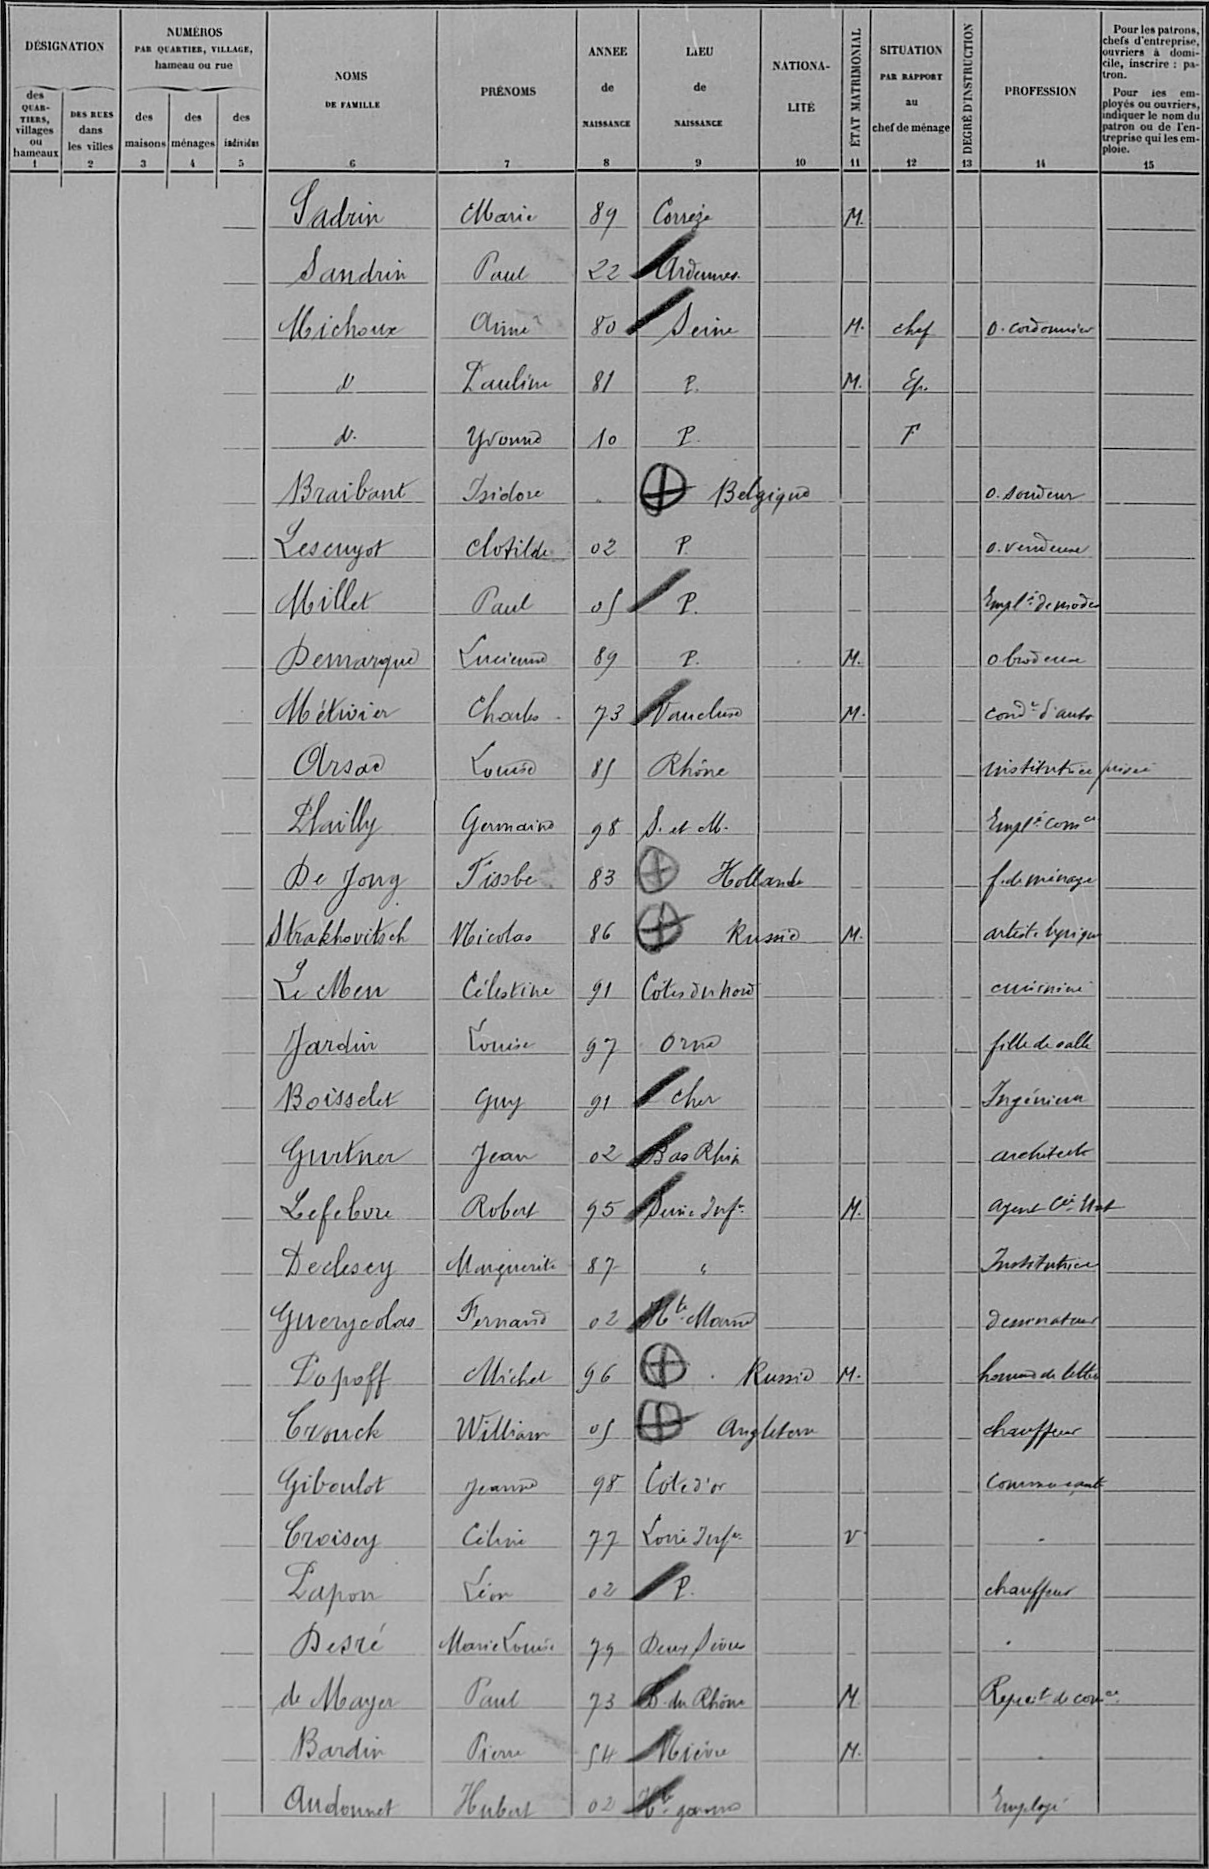Sadrin/Marie/89/Corrèze/¤/M /¤/¤/¤/¤
Sandrin/Paul/22/Ardennes/¤/¤/¤/¤/¤/¤
Michoux/Aimé/80/Seine/¤/M /chef/¤/O Cordonnier/¤
d°/Pauline/81/P /¤/M /Ep /¤/¤/¤
d°/Yvonne/10/P/¤/¤/F/¤/¤/¤
Braibant/Isidore/¤/Belgique/¤/¤/¤/¤/o soudeur/¤
Lescuyot/Clotilde/02/P/¤/¤/¤/¤/o vendeuse/¤
Millet/Paul/05/P /¤/¤/¤/¤/Empl de mode/¤
Demarque/Lucienne/89/P /¤/M /¤/¤/o brodeuse/¤
Métivier/Charles/73/Vaucluse/¤/M /¤/¤/cond d'auto/¤
Arsac/Louise/85/Rhône/¤/¤/¤/¤/institutrice privée/¤
Dhailly/Germaine/98/S et M/¤/¤/¤/¤/Emplé comce/¤
De Jong/Fissbe/83/Hollande/¤/¤/¤/¤/f de ménage/¤
Strakhovitsch/Nicolas/86/Russie/¤/M/¤/¤/artiste lyrique/¤
Le Meu/Célestine/91/Cotes du Nord/¤/¤/¤/¤/cuisinière/¤
Jardin/Louise/97/Orne/¤/¤/¤/¤/fille de salle/¤
Boisselet/Guy/91/Cher/¤/¤/¤/¤/Ingénieur/¤
Gurtner/Jean/02/Bas Rhin/¤/¤/¤/¤/architecte/¤
Lefebvre/Robert/95/Seine Inf/¤/M /¤/¤/agent Cie Nat/¤
Declesey/Marguerite/87/¤/¤/¤/¤/¤/Institutrice/¤
Guerycolas/Fernand/02/Hte Marne/¤/¤/¤/¤/dessinateur/¤
Dopoff/Michel/96/Russie/¤/M /¤/¤/homme de lettres/¤
Crouch/William/05/Angleterre/¤/¤/¤/¤/chauffeur/¤
Giboulot/Jeanne/98/Cote d'or/¤/¤/¤/¤/Commerçant/¤
Croisy/Céline/77/Loire Infr/¤/V/¤/¤/¤/¤
Lapon/Léon/02/P/¤/¤/¤/¤/chauffeur/¤
Desré/Marie Louise/79/Deux Sèvres/¤/¤/¤/¤/¤/¤
de Mayer/Paul/73/B du Rhône/¤/M/¤/¤/Rept de comce/¤
Bardin/Pierre/54/Nièvre/¤/M /¤/¤/¤/¤
Audonnet/Hubert/02/Hte Garonne/¤/¤/¤/¤/Employé/¤
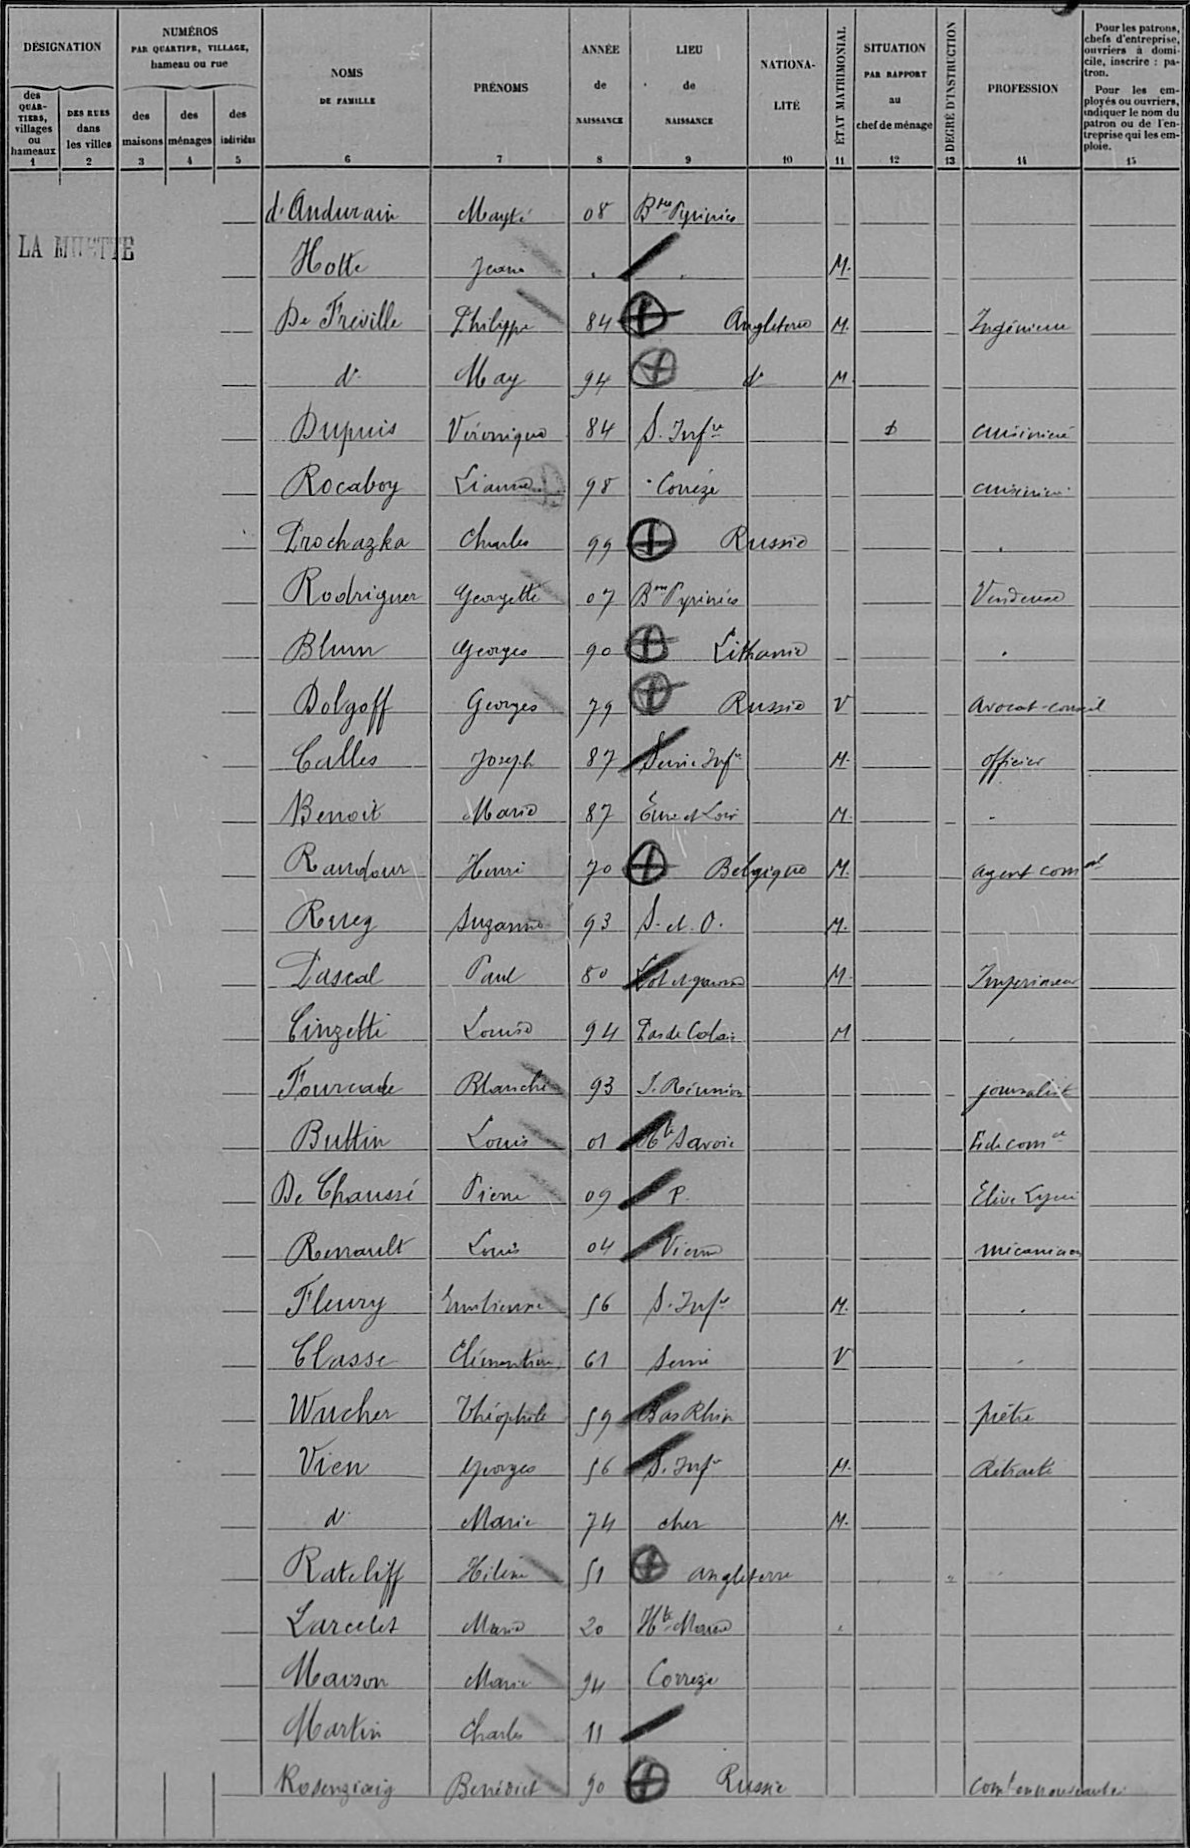d'Andurain/Mayté/08/Bse Pyrénées/¤/¤/¤/¤/¤/¤
Hotte/Jean/¤/¤/¤/M /¤/¤/¤/¤
De Freville/Philippe/84/Angleterre/¤/M /¤/¤/Ingénieur/¤
d°/May/94/d°/¤/M/¤/¤/¤/¤
Dupuis/Véronique/84/S Infre/¤/¤/D/¤/cuisinière/¤
Rocaboy/Lianne/98/Corrèze/¤/¤/¤/¤/cuisinière/¤
Drochazka/Charles/99/Russie/¤/¤/¤/¤/¤/¤
Rodriguer/Georgette/07/Bse Pyrénées/¤/¤/¤/¤/Vendeuse/¤
Blum/Georges/90/Lithuanie/¤/¤/¤/¤/¤/¤
Dolgoff/Georges/79/Russie/¤/V/¤/¤/avocat conseil/¤
Calles/Joseph/87/Seine Inf/¤/M/¤/¤/officier/¤
Benoit/Marie/87/Eure et Loir/¤/M/¤/¤/¤/¤
Randour/Henri/70/Belgique/¤/M/¤/¤/agent comal/¤
Roareg/Suzanne/93/S et O/¤/M /¤/¤/¤/¤
Daseal/Paul/80/Lot et Garonne/¤/M/¤/¤/Imprimeur/¤
Cinzetti/Louise/94/Pas de Calais/¤/M/¤/¤/¤/¤
Fourcade/Blanche/93/I Réunion/¤/¤/¤/¤/journaliste/¤
Buttin/Louis/01/Hte Savoie/¤/¤/¤/¤/E de comce/¤
De Chaussé/Pierre/09/P/¤/¤/¤/¤/Elève Lycée/¤
Renault/Louis/04/Vienne/¤/¤/¤/¤/mécanicien/¤
Fleury/Emilienne/56/S Inf/¤/M /¤/¤/¤/¤
Classe/Clémentine/61/Seine/¤/V/¤/¤/¤/¤
Wucher/Théophile/59/Bas Rhin/¤/¤/¤/¤/prêtre/¤
Vien/Georges/56/S Inf/¤/M /¤/¤/Retraite/¤
d/Marie/74/cher/¤/M /¤/¤/¤/¤
Rateliff/Hilen/51/angleterre/¤/¤/¤/¤/¤/¤
Larcelet/Marie/20/Hte Marne/¤/¤/¤/¤/¤/¤
Maison/Marie/94/Corrèze/¤/¤/¤/¤/¤/¤
Martin/Charles/11/¤/¤/¤/¤/¤/¤/¤
Rosengixig/Benedict/90/Russie/¤/¤/¤/¤/couturière en nouveauté/¤